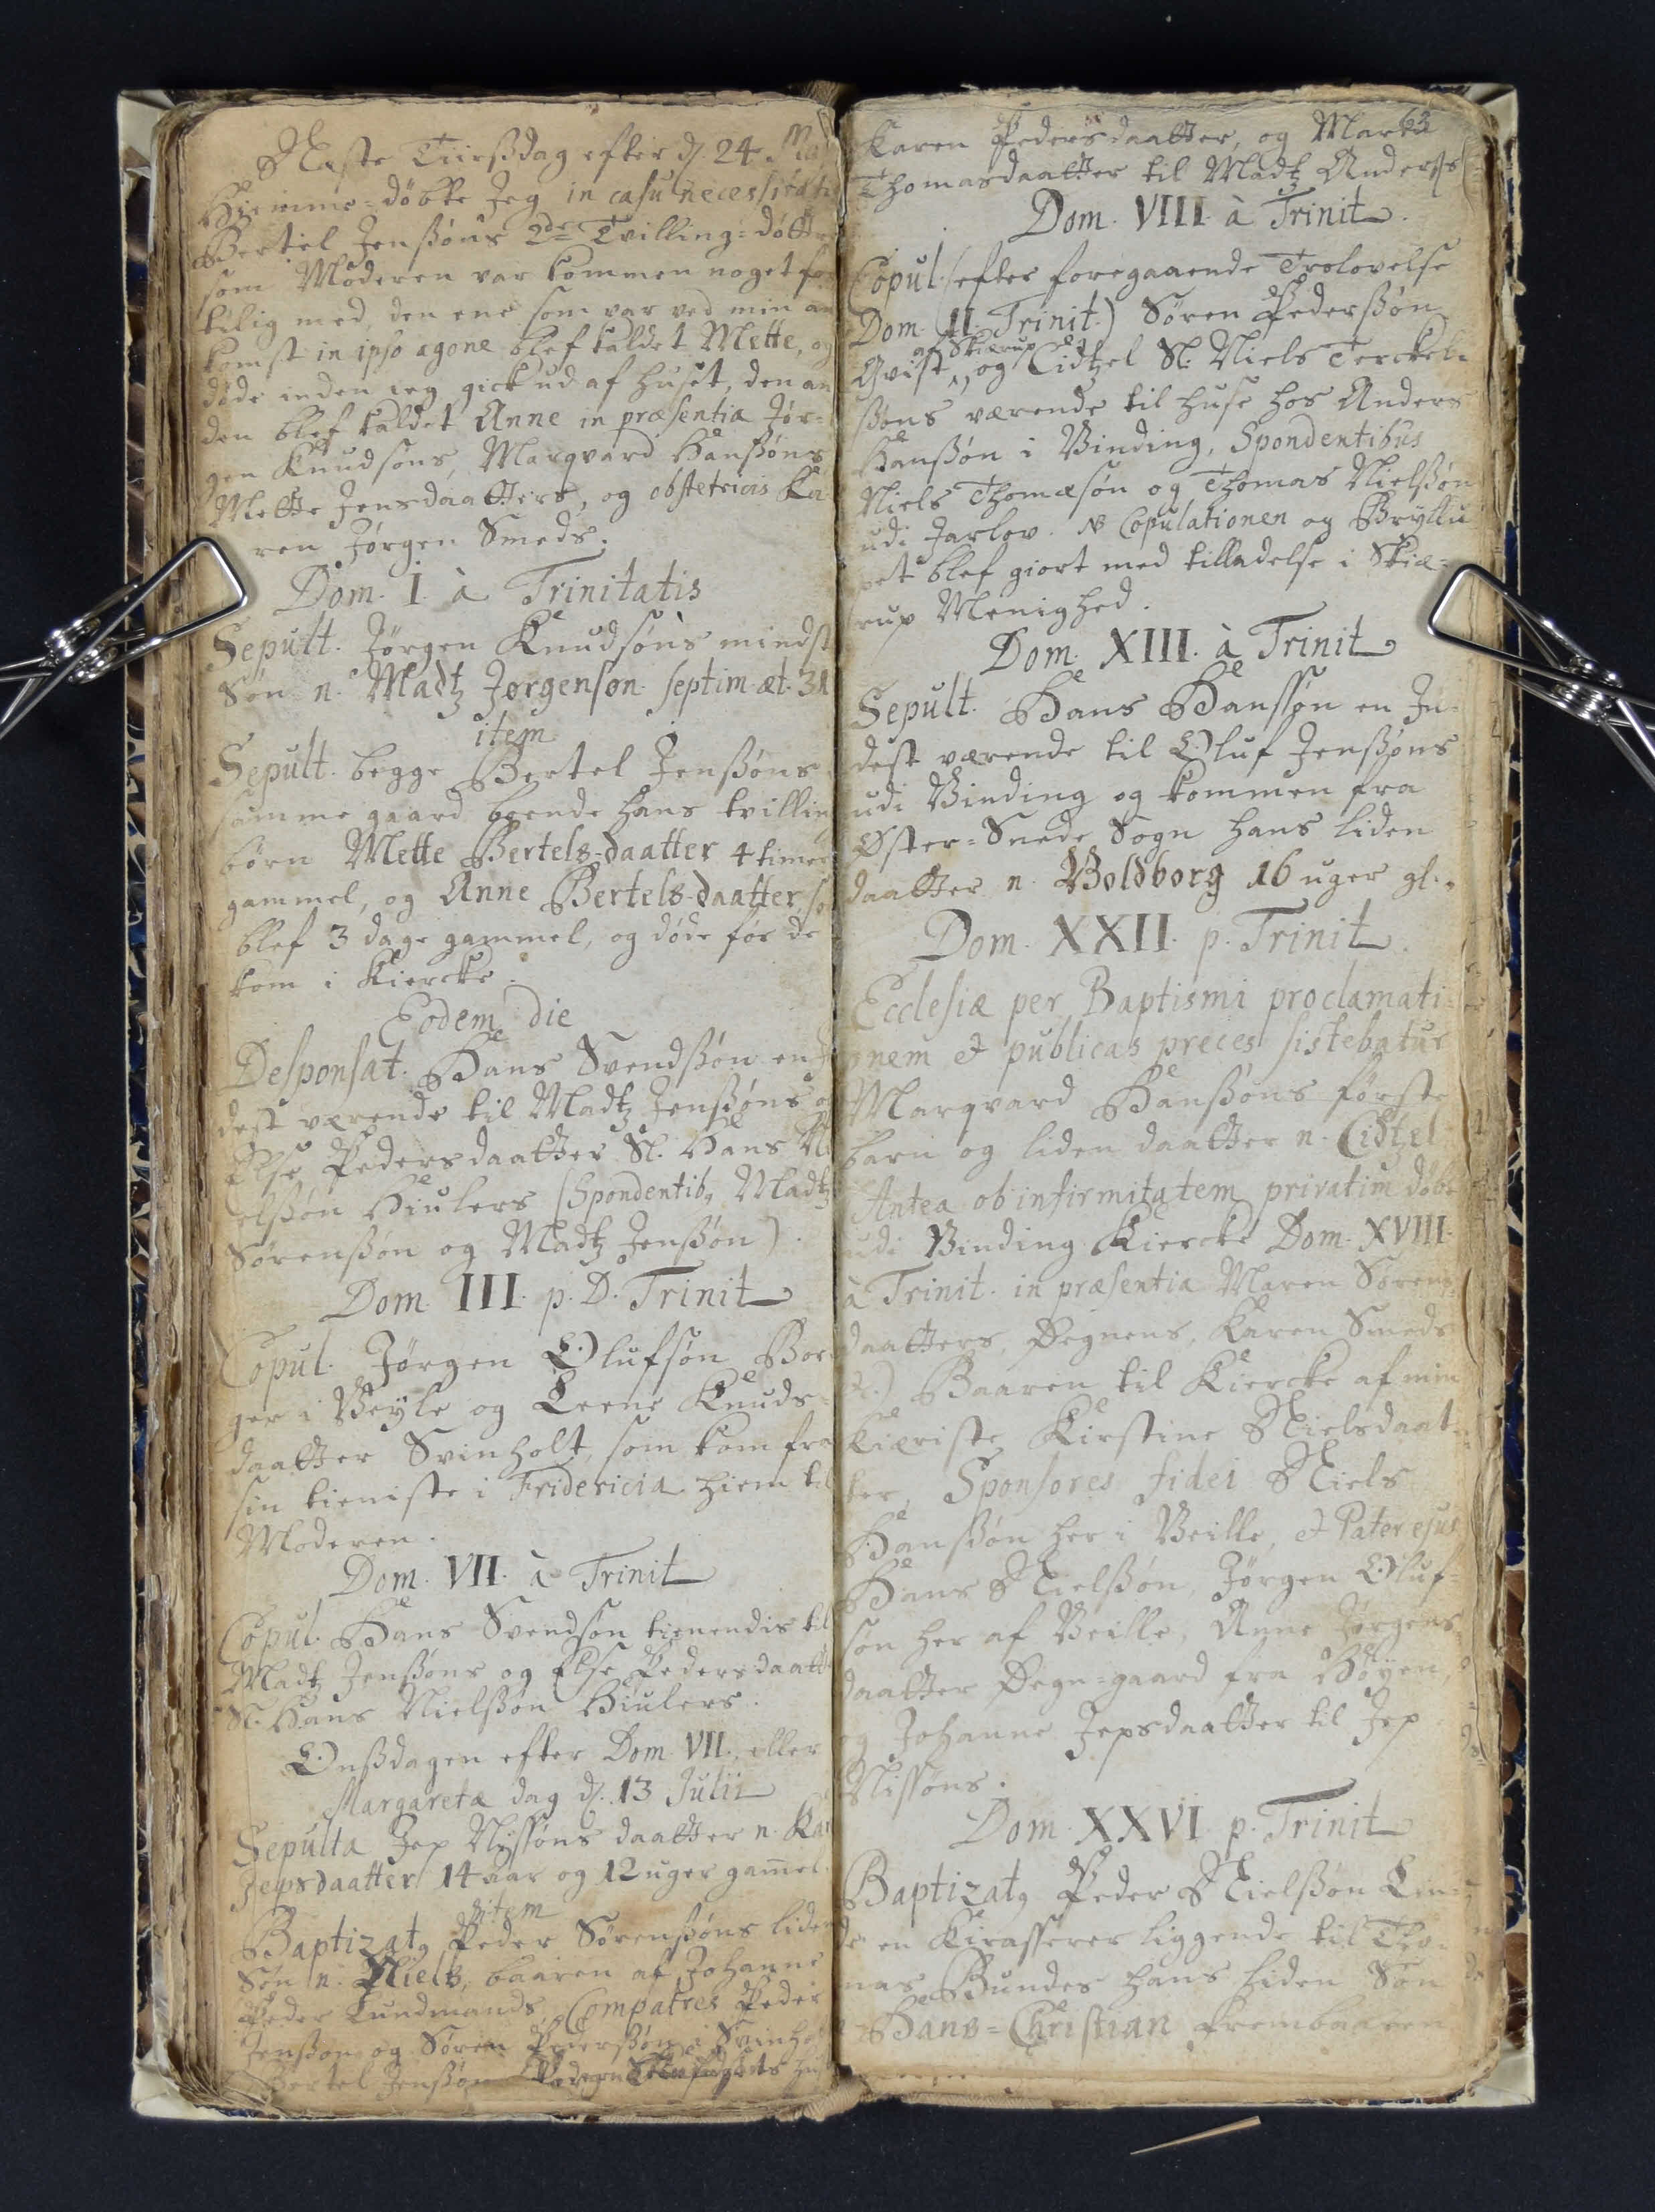Næste Tiirssdag efter d. 24 Ma 
Hiemme=døbte Jeg in casu## necessit##
Bertel Jenssøns 2de Tvilling=døtre
som Moderen var kommen noget for
tidlig med, den ene som var ved min an
komst in ipso agone blef kaldet Mette, og 
døde inden ieg gik ud af huset, den an
den blef kaldet Anne in præsentia Jør¬
gen Knudsøns, Marqvard Hanssøns
Mette Jensdaatters, og obstetricis Ka¬
ren Jørgen Smeds
Dom. I. a Trinit
Sepult. Jørgen Knudsøns mindste
Søn n. Madtz Jørgensøn septim æt. 31
item
Sepult. begge Bertel Jenssøns
samme gaard boende hans tvilling 
børn Mette Bertels-daatter 4 timer
gammel, og Anne Bertels-daatter, so 
blef 3 dage gammel, og døde før de
kom i Kiercke
Eodem die
Desponsat. Hans Svendssøn en
derst værende til Madtz Jenssøns og
Else Pedersdaatter Sl. Hans Ni
elssøn Hiulers (Spondentib Madtz
Sørenssøn og Madtz Jenssøn).
Dom. III. p. D. Trinit
Copul. Jørgen Olufsøn Bor¬
ger i Veyle og Leene Knuds¬
daatter Svinholt, som kom fra
sin tieneste i Fridericia hiem til
Moderen
Dom. VII. a Trinit
Copul Hans Svendsøn tienendis til
Madtz Jenssøns og Else Pedersdaatte 
Sl. Hans Nielssøn Hiulers
Onssdagen efter Dom. VII. eller
Margaretæ dag d. 13 Julii
Sepulta Jep Nissøns daatter n. Kar 
Jepsdaatter 14 aar og 12 uger gammel.
Item
Baptizatq Peder Sørensøns liden
Søn n. Niels, baaren af Johanne
Peder Lundmands, Compatres Peder
Jenssøn og Søren Pederssøn i Svinholt
Bertel Jenssøn ## Skovfogets hust 
63
Karen Pedersdaatter, og Maren
Thomasdaatter til Madtz Anders
Dom. VIII. a Trinit.
Copul. (efter foregaaende Trolovelse
Dom. II Trinit.) Søren Pederssøn
af Skiærup
Qvist og Cidtzel Sl. Niels Terckel¬
ssøns værende til huse hos Anders
Hanssøn i Vinding, Spondentibus
Niels Thomæsøn og Thomas Nielssøn
udi Jarlow. NB Copulationen og Bryllu 
pet blef giort med tilladelse i Skiæ¬
rup Menighed
Dom. XIII. a Trinit
Sepult. Hans Hansøn en In¬
derst værende til Oluf Jenssøns
udi Vinding og kommen fra
Øster=Snede Sogn, hans liden
daatter n. Voldborg 16 uger gl.
Dom. XXII. p. Trinit
Ecclesiæ per Baptismi proclamati¬
onem et publicas preces sistebatur
Marqvard Hanssøns første
barn og liden daatter n. Cidtzel
Antea obinfirmitatem privatim døbt 
udi Vinding Kiercke Dom. XVIII.
a Trinit. in præsentia Maren Sørens¬
daatter Degnens, Karen Smeds
Baaren til Kiercke af min
Kiæriste Kirstine Nielsdaat¬
ter, Sponsores didei Niels
Hanssøn her i Veille. et Pater ejus
Hans Nielssøn, Jørgen Oluf¬
søn her af Veille, Anne Jørgens¬
daatter Degn=gaard fra Høyen,
og Johanne Jepsdaatter til Jep
Nissøns
Dom. XXVI p. Trinit
Baptizatq Peder Nielssøn
Kirasserer liggende til Tho¬
mas Bundes hans liden Søn
Hans=Christian, frembaaren